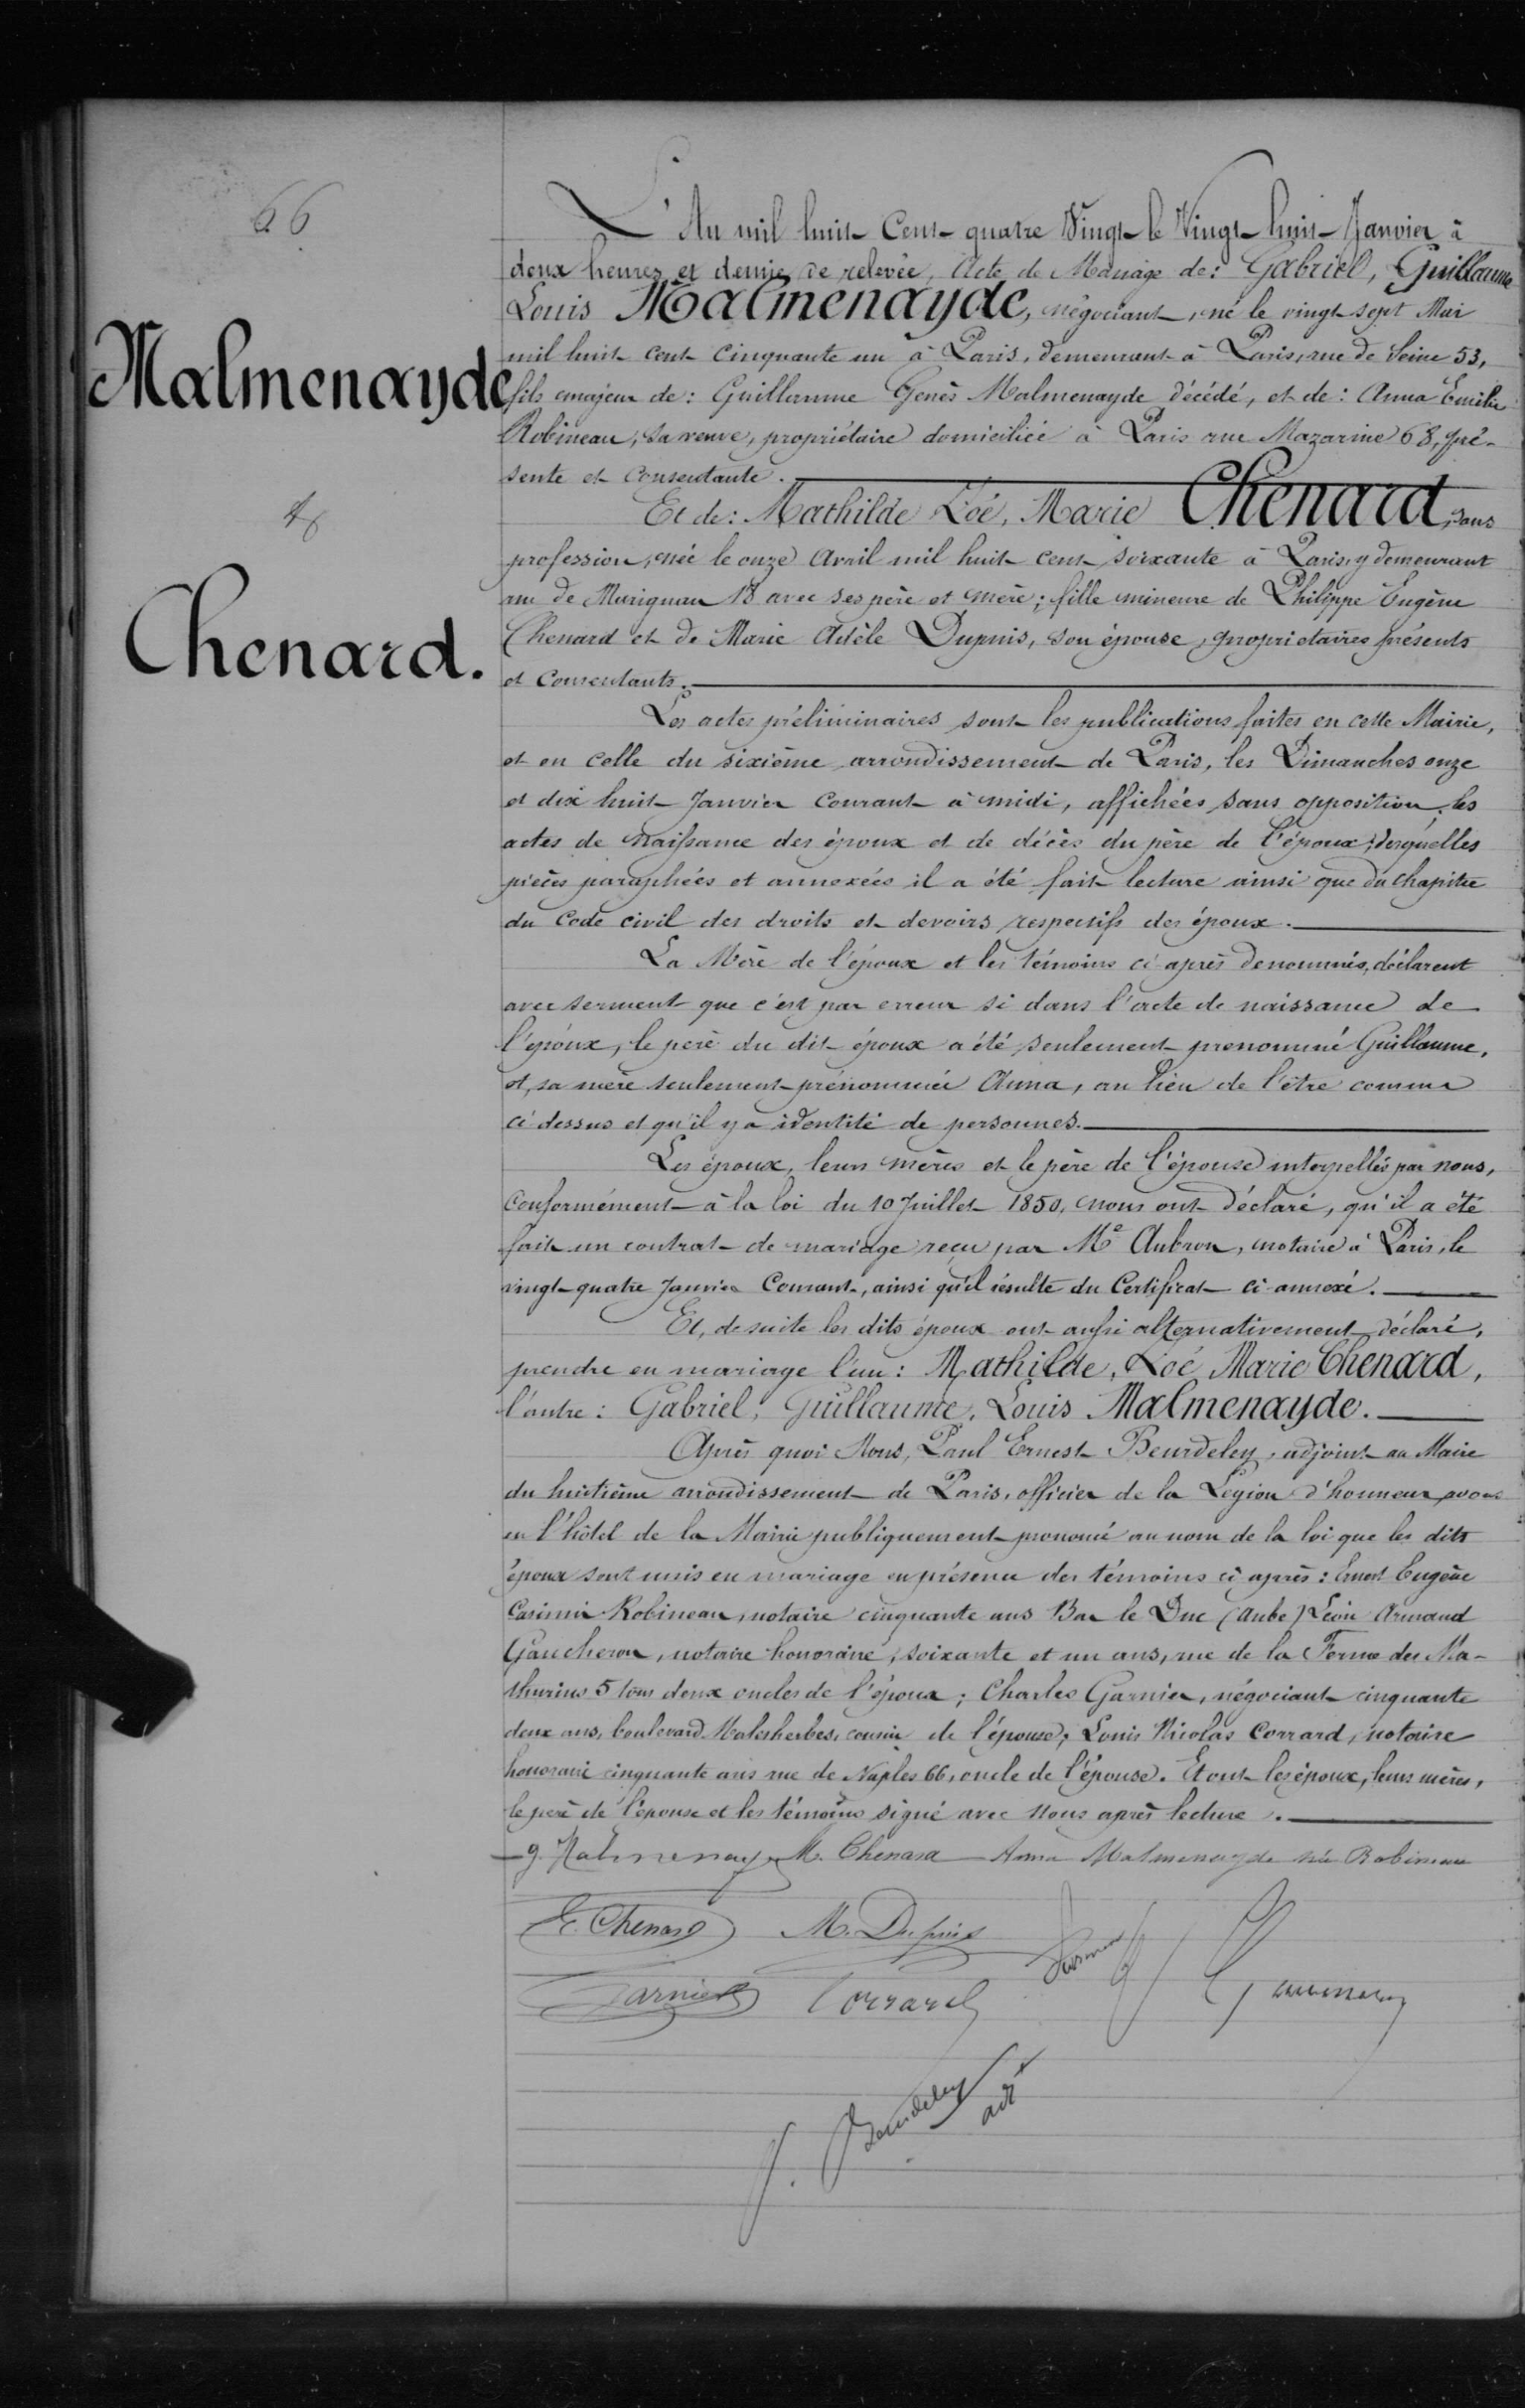66
Malmenayde
&
Chenard
L'An mil huit-cent-quatre Vingt le Vingt huit Janvier à
deux heures et demie de relevée, Acte de Mariage de: Gabriel, Guillaume
Louis Malmenayde, négociant, né le vingt sept Mai
mil huit cent cinquante un à Paris, demeurant à Paris, rue de Seine 53,
fils majeur de: Guillaume Genès Malmenayde décédé, et de: Anna Emilie
Robineau, sa veuve, propriétaire domiciliée à Paris rue Mazarine 68, pré-
sente et consentante. -
Et de: Mathilde Zoé, Marie, Chénard, sans
profession, née le onze avril mil huit cent soixante à Paris, y demeurant
rue de Marignan 18 avec ses père et mère; fille mineure de Philippe Eugène
Chenard et de Marie Adèle Dupuis, son épouse, propriétaires présents
et consentants. -
Les actes préliminaires sont les publications faites en cette Mairie,
et en celle du sixième arrondissement de Paris, les Dimanches onze
et dix huit janvier courant à midi, affichées sans opposition les
actes de naissance des époux et de décès du père de l'époux. Lesquelles
pièces paraphées et annexées il a été fait lecture ainsi que du chapitre
du Code civil des droits et devoirs respectifs des époux. -
La Mère de l'époux et les témoins ci après dénommés, déclarent
avec serment que c'est par erreur si dans l'acte de naissance de
l'époux, le père du dit époux a été seulement prénommé Guillaume,
et, sa mère seulement prénommée Anna, au lieu de l'être connu
ci-dessus et qu'il y a identité de personnes. -
Les époux, leurs mères et le père de l'épouse interpellée par nous,
conformément à la loi du 10 Juillet 1850, nous ont déclaré, qu'il a été
fait un contrat de mariage reçu par Me Aubron, notaire à Paris, le
vingt-quatre Janvier courant, ainsi qu'il résulte du Certificat ci-annexé. -
Et, de suite les dits époux ont aussi alternativement déclaré,
prendre en mariage l'un: Mathilde, Zoé, Marie Chenard,
l'autre: Gabriel, Guillaume, Louis, Malmenayde. -
Après quoi Nous, Paul Ernest Beurdeley, adjoint au Maire
du huitième arrondissement de Paris, officier de la Légion d'honneur, avons
en l'hôtel de la Mairie publiquement prononcé au nom de la loi que les dits
époux sont unis en mariage en présence des témoins ci après: Ernest Eugène
Casimir Robinson notaire cinquante ans Bar le Duc (Aube) Léon Arnaud
Gaucheron, notaire honoraire, soixante et un ans, rue de la Ferme des Ma-
thurins 5 tous deux oncles de l'époux; Charles Garnier, négociant cinquante
deux ans, boulevard Malsherbes, cousin de l'épouse, Louis Nicolas Corrard, notaire
honoraire cinquante ans rue de Naples 66, oncle de l'épous. Et ont les époux, leurs mères,
le père de l'époux et les témoins signé avec nous après lecture. -
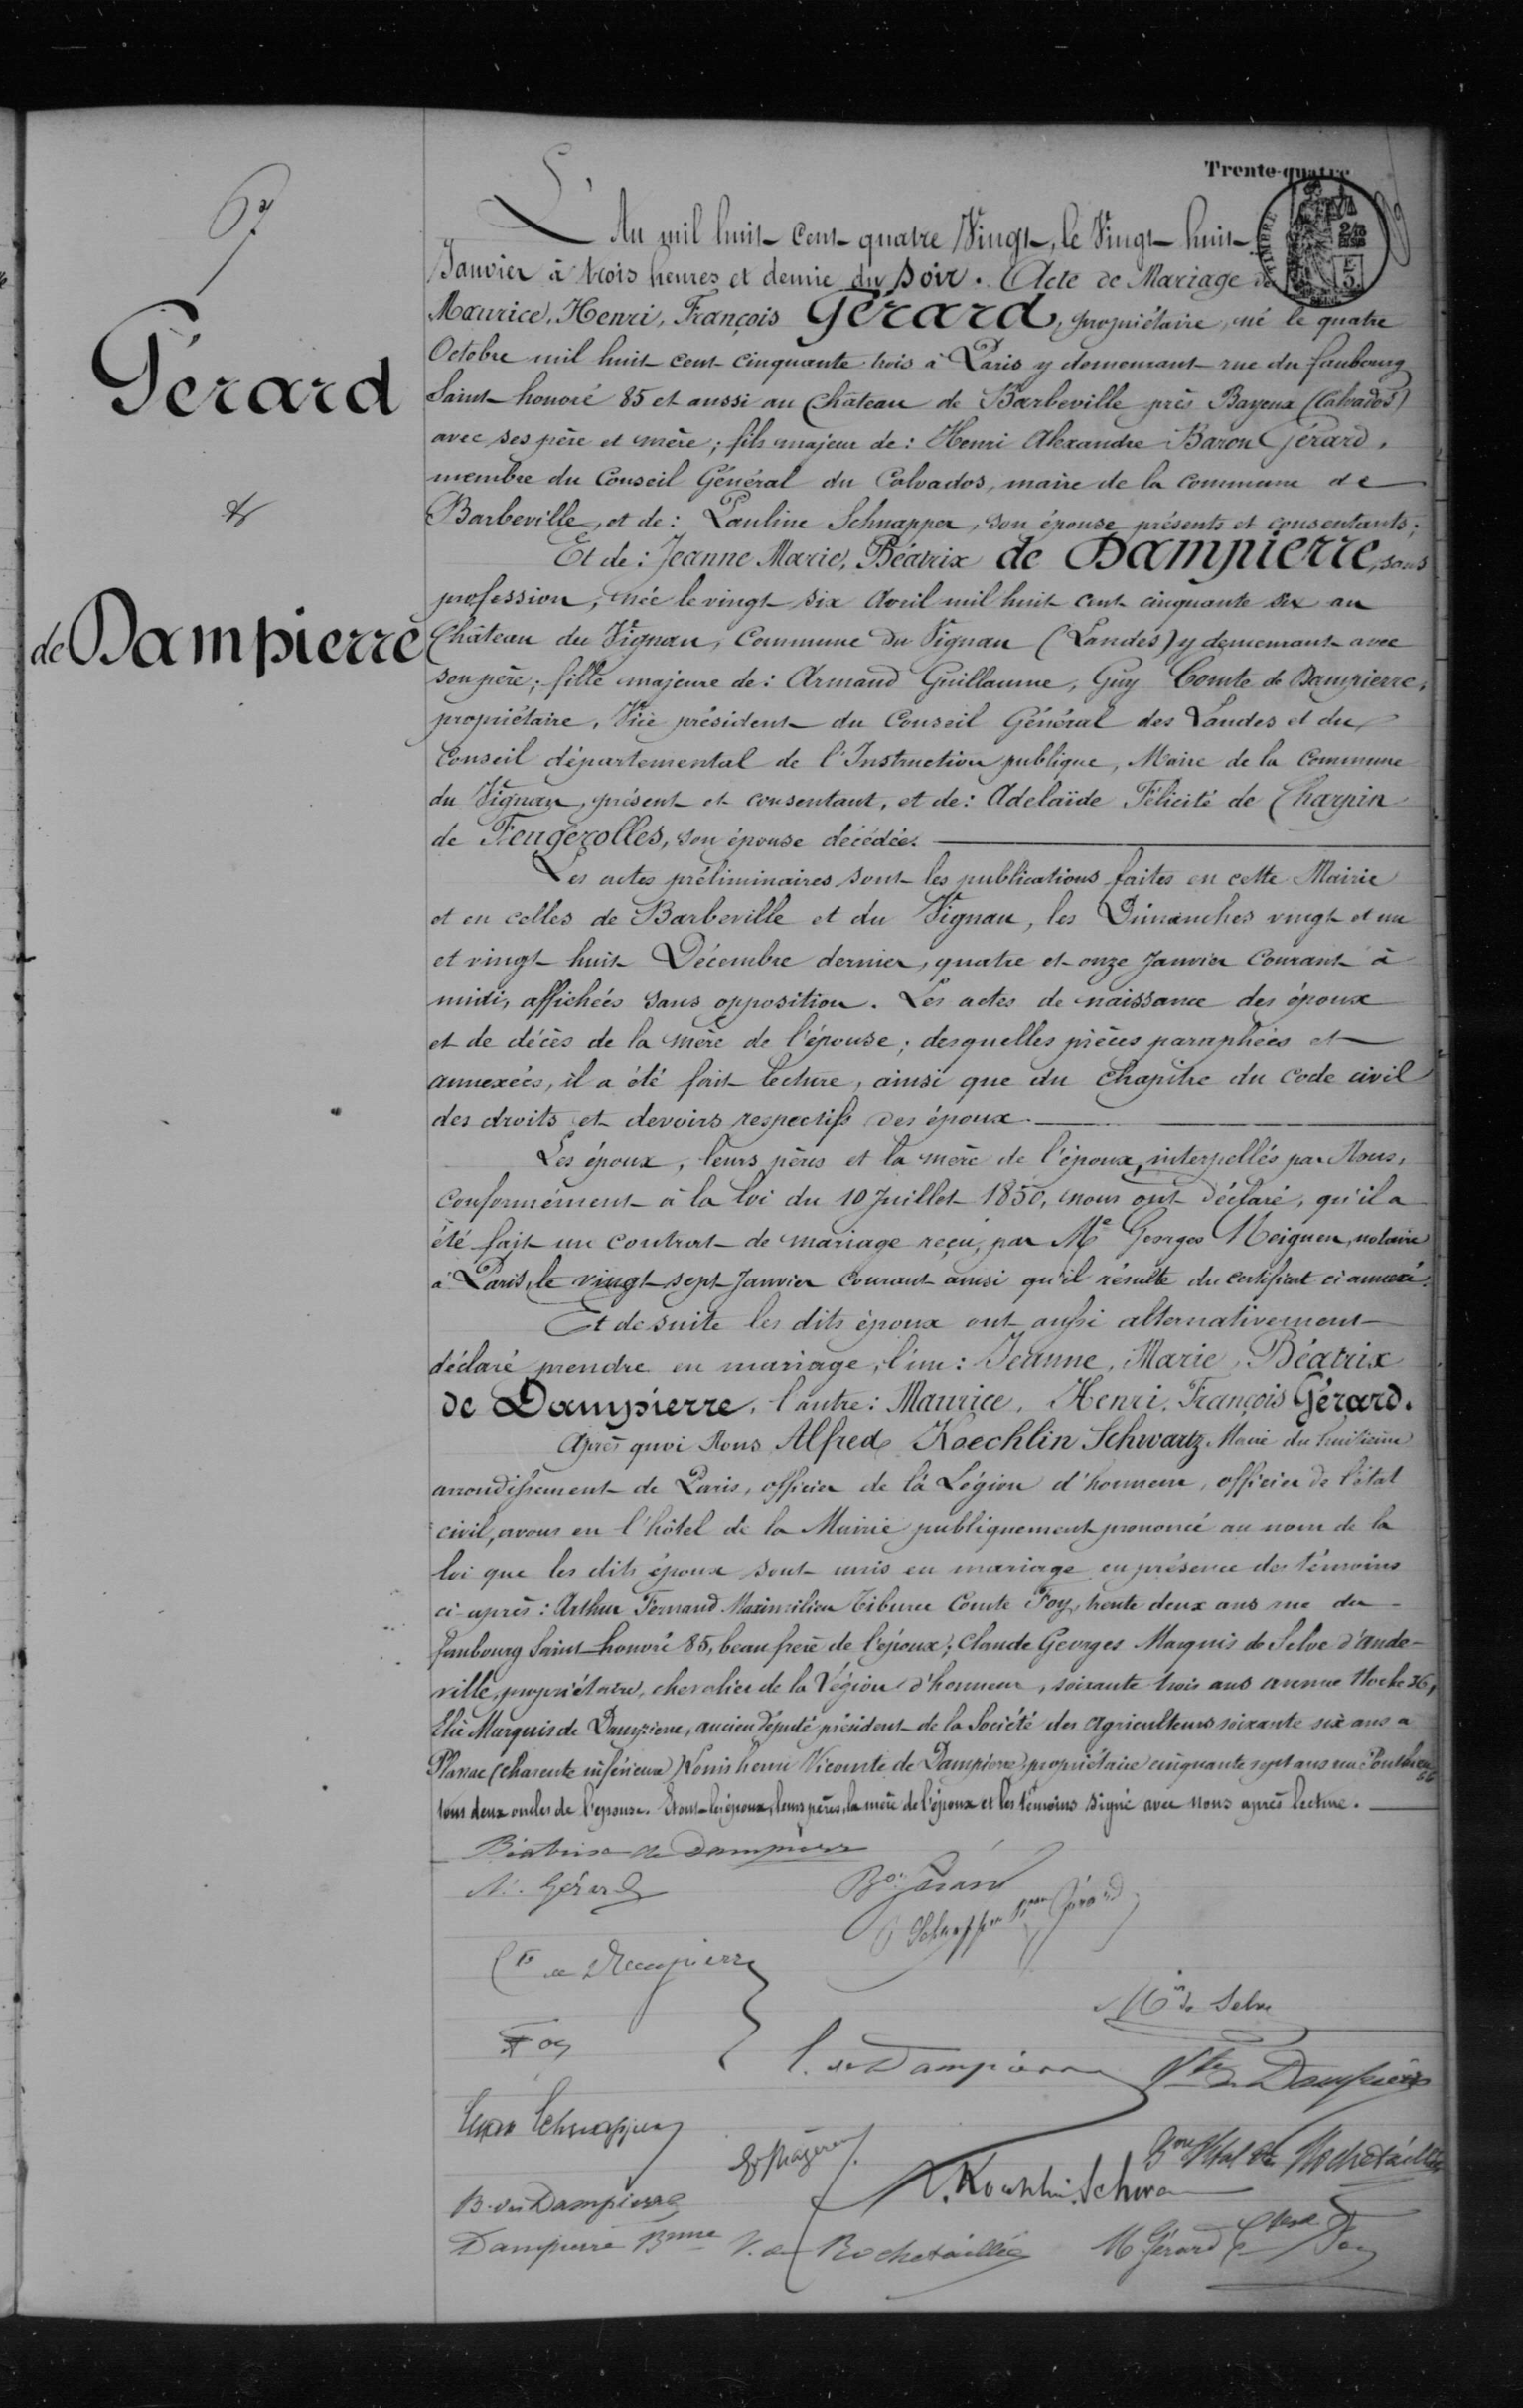67
Gérard
&
de Dampierre
L'An mil huit cent-quatre-vingt-, le Vingt-huit-
Janvier à trois heures et demie du soir. Acte de Mariage de
Maurice, Henri, François Gérard, propriétaire, né le quatre
Octobre mil huit cent cinquante trois à Paris y demeurant rue du faubourg
Saint-honoré 85 et aussi au château de Barbeville près Bayeux (Calvados)
avec ses père et mère; fils majeur de: Henri Alexandre Barou Gérard,
membre du Conseil Général du Calvados, maire de la commune de
Barbeville, et de: Pauline Schmapper, son épouse présents et consentants;
Et de: Jeanne Marie, Béatrice de Dampierre, sans
profession, née le vingt-six août mil huit cent cinquante six au
Château du Vignan, Commune du Vignan (Landes) y demeurant avec
son père; fille majeure de: Arnaud Guillaume, Guy Comte de Dampierre,
propriétaire, Vice président du Conseil Général des Landes et du
Conseil départemental de l'Instruction publique, Maire de la commune
du Vignan, présent et consentant, et de: Adelaïde Félicité de Charpin
de Fengerolles, son épouse décédée. -
Les actes préliminaires sont les publications faites en cette Mairie
en celles de Barbeville et du Vignan, les Dimanches vingt et un
et vingt huit Décembre dernier, quatre et onze Janvier courant à
midi, affichées sans opposition. Les actes de naissance des époux
et de décès de la mère de l'épouse; desquelles pièces paraphées et
annexées, il a été fait lecture, ainsi que du chapitre du code civil
des droits et devoirs respectifs des époux. -
Les époux, leurs pères et la mère de l'époux, interpellés par Nous,
conformément à la Loi du 10 Juillet 1850, nous ont déclaré, qu'il a
été fait un contrat de mariage reçu par Me Georges Meignen, notaire
à Paris, le vingt sept Janvier courant ainsi qu'il résulte du certificat ci annexé
Et de suite les dits époux ont aussi alternativement
déclaré prendre en mariage, l'un: Jeanne, Marie, Béatrix
de Dampierre, l'autre: Maurice, Henri, François Gérard.
Après quoi Nous Alfred Koechlin Schwartz, Maire du huitième
arrondissement de Paris, officier de la Légion d'honneur, officier de l'état
civil, avons en l'hôtel de la Mairie publiquement prononcé au nom de la
loi que les dits époux sont unis en mariage en présence des témoins
ci-après: Arthur Fernand Maximilien Tiburce Comte Foy, trente deux ans rue du
faubourg Saint honoré 85, beau-frère de l'époux; Claude Georges Marquis de Seluc d'Ande-
ville, propriétaire, chevalier de la Légion d'honneur, soixante-trois ans avenue Hocke 36,
Elie Marquis de Dampierre, ancien député président de la Société des agriculteurs soixante six ans a
Plassac (charente inférieure) Louis henri Vicomte de Dampierre, propriétaire cinquante sept ans rue Ponthieu 56
tous deux oncles de l'épouse. Et ont les époux, leurs pères, la mère de l'époux et les témoins signé avec nous après lecture. -